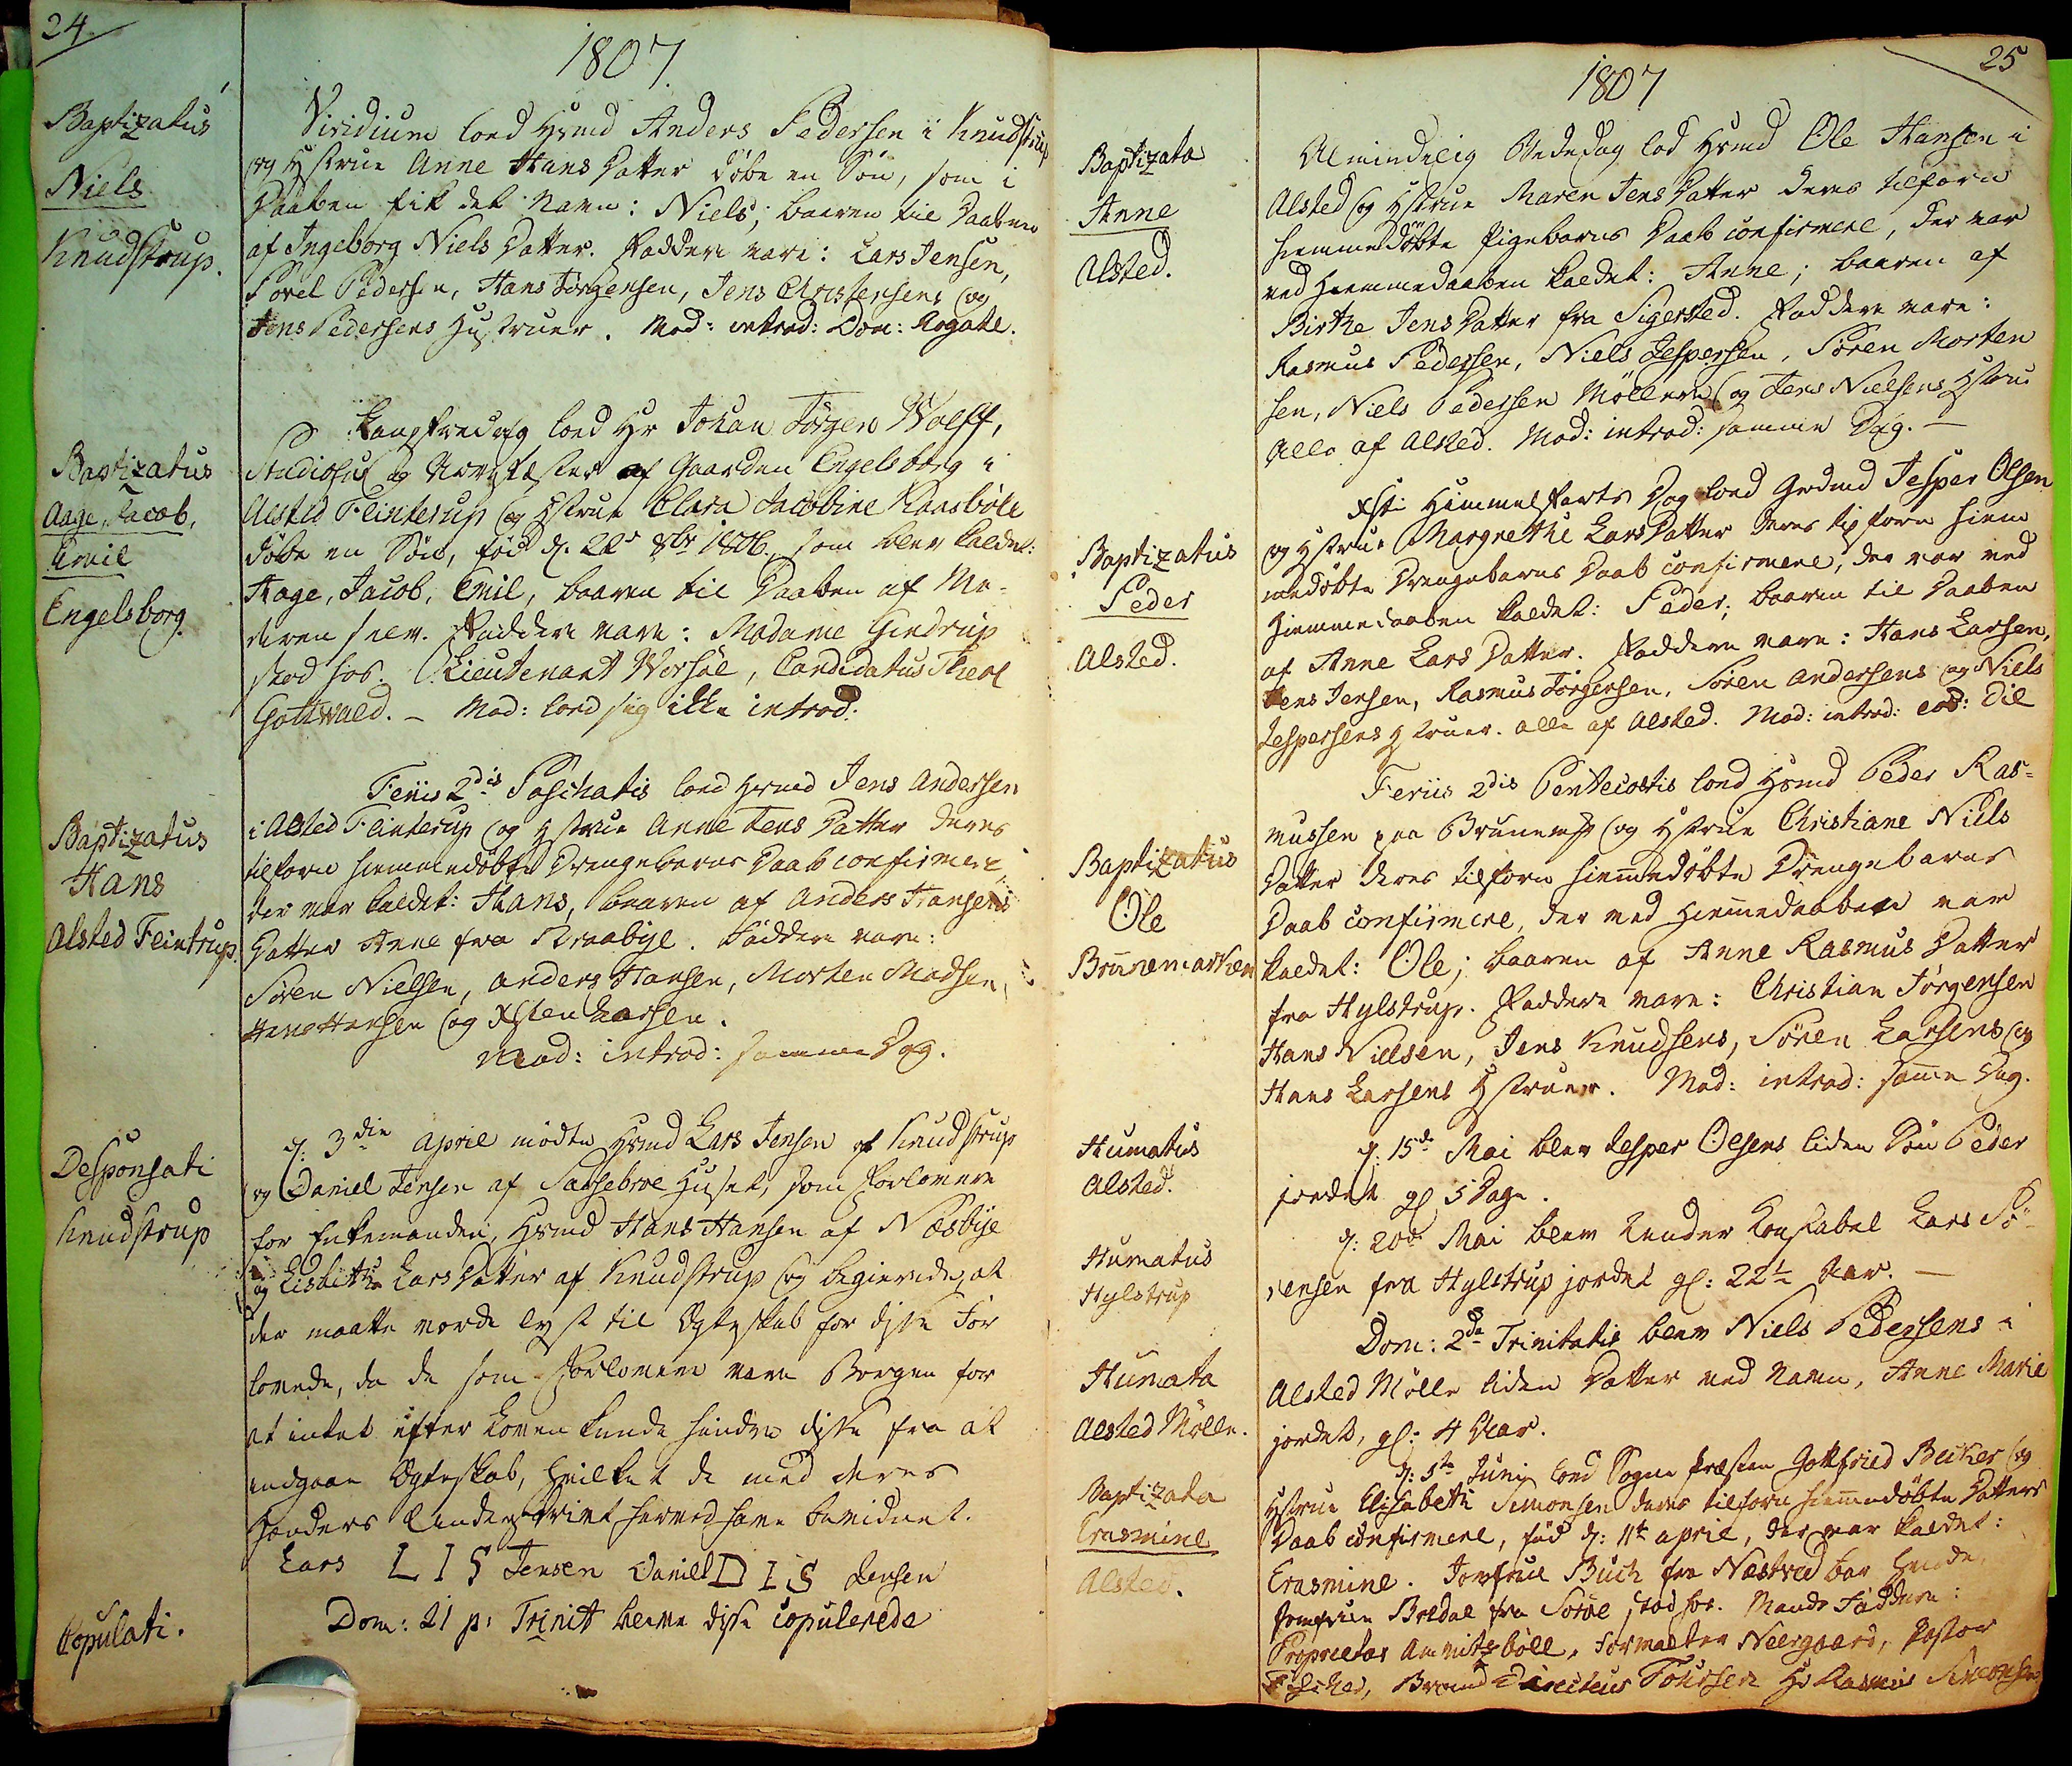24.
Baptizatus
Niels
Knudstrup.
Baptizatus
Aage Jacob
Emil
Engelsborg
Baptizatus
Hans
Alsted Flinterup.
Desponsati
Knudstrup
Copulati.
1807.
Viridium loed Hsmd Anders Pedersen i Knudstrup
og Hstrue Anne Hans Datter døbe en Søn, som i
Daaben fik det Navn: Niels; baaren til Daaben
af Ingeborg Niels Datter. Faddere vare: Lars Jensen,
Povel Pedersen, Hans Jørgensen, Jens Christensens og
Jens Pedersens Hustruer. Mod: introd: Dom: Rogate.
Langfredag loed Hr Johan Jørgen Wolff,
Studiosus og Arvefæsterr af Gaarden Engelsborg i
Alsted Flinterup og Hustrue Clara Jacobine Kaasbøle
døbe en Søn, fød d: 20de 8br 1806, som blev kaldet:
Aage, Jacob Emil, baaren til Daaben af Mo¬
deren selv. Faddere vare: Madame Gindrup
stod hos. Lientenant Wersøe, Candidatus Theol
Gottwald. - Mod: loed sig ikke introd:
Feriis 2dis Paschatis loed Hsmd Jens Andersen
i Alsted Flinterup og Hstrue Anne Jens Datter deres
tilforn hiemmedøbte Drengebarns Daab confirmere
der var kaldet: Hans, baaren af Anders Hansens
Datter Anne fra Braabye. Faddere vare:
Søren Nielsen, Anders Hansen, Morten Madsen
Hans Hansen og Xsten Larsen.
Mod: introd: samme Dag.
d: 3die April mødet Hsmd Lars Jensen af Knudstrup
og Daniel Jensen af Sa##sebroe Huset, som Forlovere
for Enkemanden, Hsmd Hans Hansen af Næsbye
og Lisbeth Lars Datter af Knudstrup og begierede, at
der maatte vorde lyst til Ægteskab for disse For
lovede, da de som Forlovere vare Borgen for
at intet efter Konen kunde hindre disse fra at
indgaare Ægteskab, hvilket de med deres
Hænders Underskrivt herved have bevidnet.
Lars L I S Jensen Daniel D I S Jensen
Dom: 21 p: Trinit bleve disse copulerede
Baptizata
Anne
Alsted.
Baptizatus
Peder
Alsted.
Baptizatus
Ole
Brunemarken
Humatus
Alsted.
Humatus
Hylstrup
Humata
Alsted Mølle.
Baptizata
Erasmine
Alsted.
25.
1807
Almindelig Bededag lod Hsmd Ole Hansen i
Alsted og Hustrue Maren Jens Datter deres tilforn
hiemmedøbte Pigebarns Daab confirmere, der var
ved Hiemmedaaben kaldet: Anne; baaren af
Birthe Jens Datter fra Sigersted. Faddere vare:
Rasmus Pedersen, Niels Jespersen, Søren Morten
sen, Niels Pedersen Mølleren og Jens Nielsens Hstrue
Alle af Alslev. Mod: introd: samme Dag.
Xsti Himmelfarts Dag loed Grdmd Jesper Olsen
og Hstrue Margrethe Lars Datter deres tilforn hiem
medøbte Drengebarns Daab confirmere, der var ved
Hiemmedaaben kaldet: Peder; baaren til Daaben
af Anne Lars Datter. Faddere vare: Hans Larsen,
Jens Jensen, Rasmus Jørgensen, Søren Andersens og Niels
Jespersens Hstruer. alle af Alsted. Mod: introd: eod: Die
Feriis 2dis Pentecostis loed Hsmd Peder Ras¬
mussen paa Brunem## og Hstrue Christiane Niels
Datter deres tilforn hiemmedøbte Drengebarns
Daab confirmere, der ved Hiemmedaaben var
kaldet: Ole; baaren af Anne Rasmus Datter
fra Hylstrup. Faddere vare: Christian Jørgensen
Hans Nielsen, Jens Knudsens, Søren Larsens og
Hans Larsens Hstruer. Mod: introd: samme Dag.
d; 15de Mai blev Jesper Olsens liden Søn Peder
joder gl 5 Dage.
d: 20de Mai blev Under Konstabel Lars Sø¬
rensen fra Hylstrup jordet gl: 22½ Aar.
Dom: 2da Trinitatis blev Niels Pedersens i
Alsted Mølle liden Datter ved Navn, Anne Marie
jordet gl: 4 Aar.
d: 5te Juni loed Sogne Præsten Gottfried Becher og
Hstrue Elisabeth Simonsen deres tilforn hiemmedøbte Datters
Daab confirmere, fød d: 11te April, der var kaldet:
Erasmine. Jomfrue Buch fra Næstved bar hende
Jomfrue Bodel fra Sorøe stod hos.l. Mands Faddere:
Proprietar Amnitzbøll, Forvalter Neergaard, Pastor
Fischer, Br## Directeur Tohrsen Hr Rasmus Simonsen
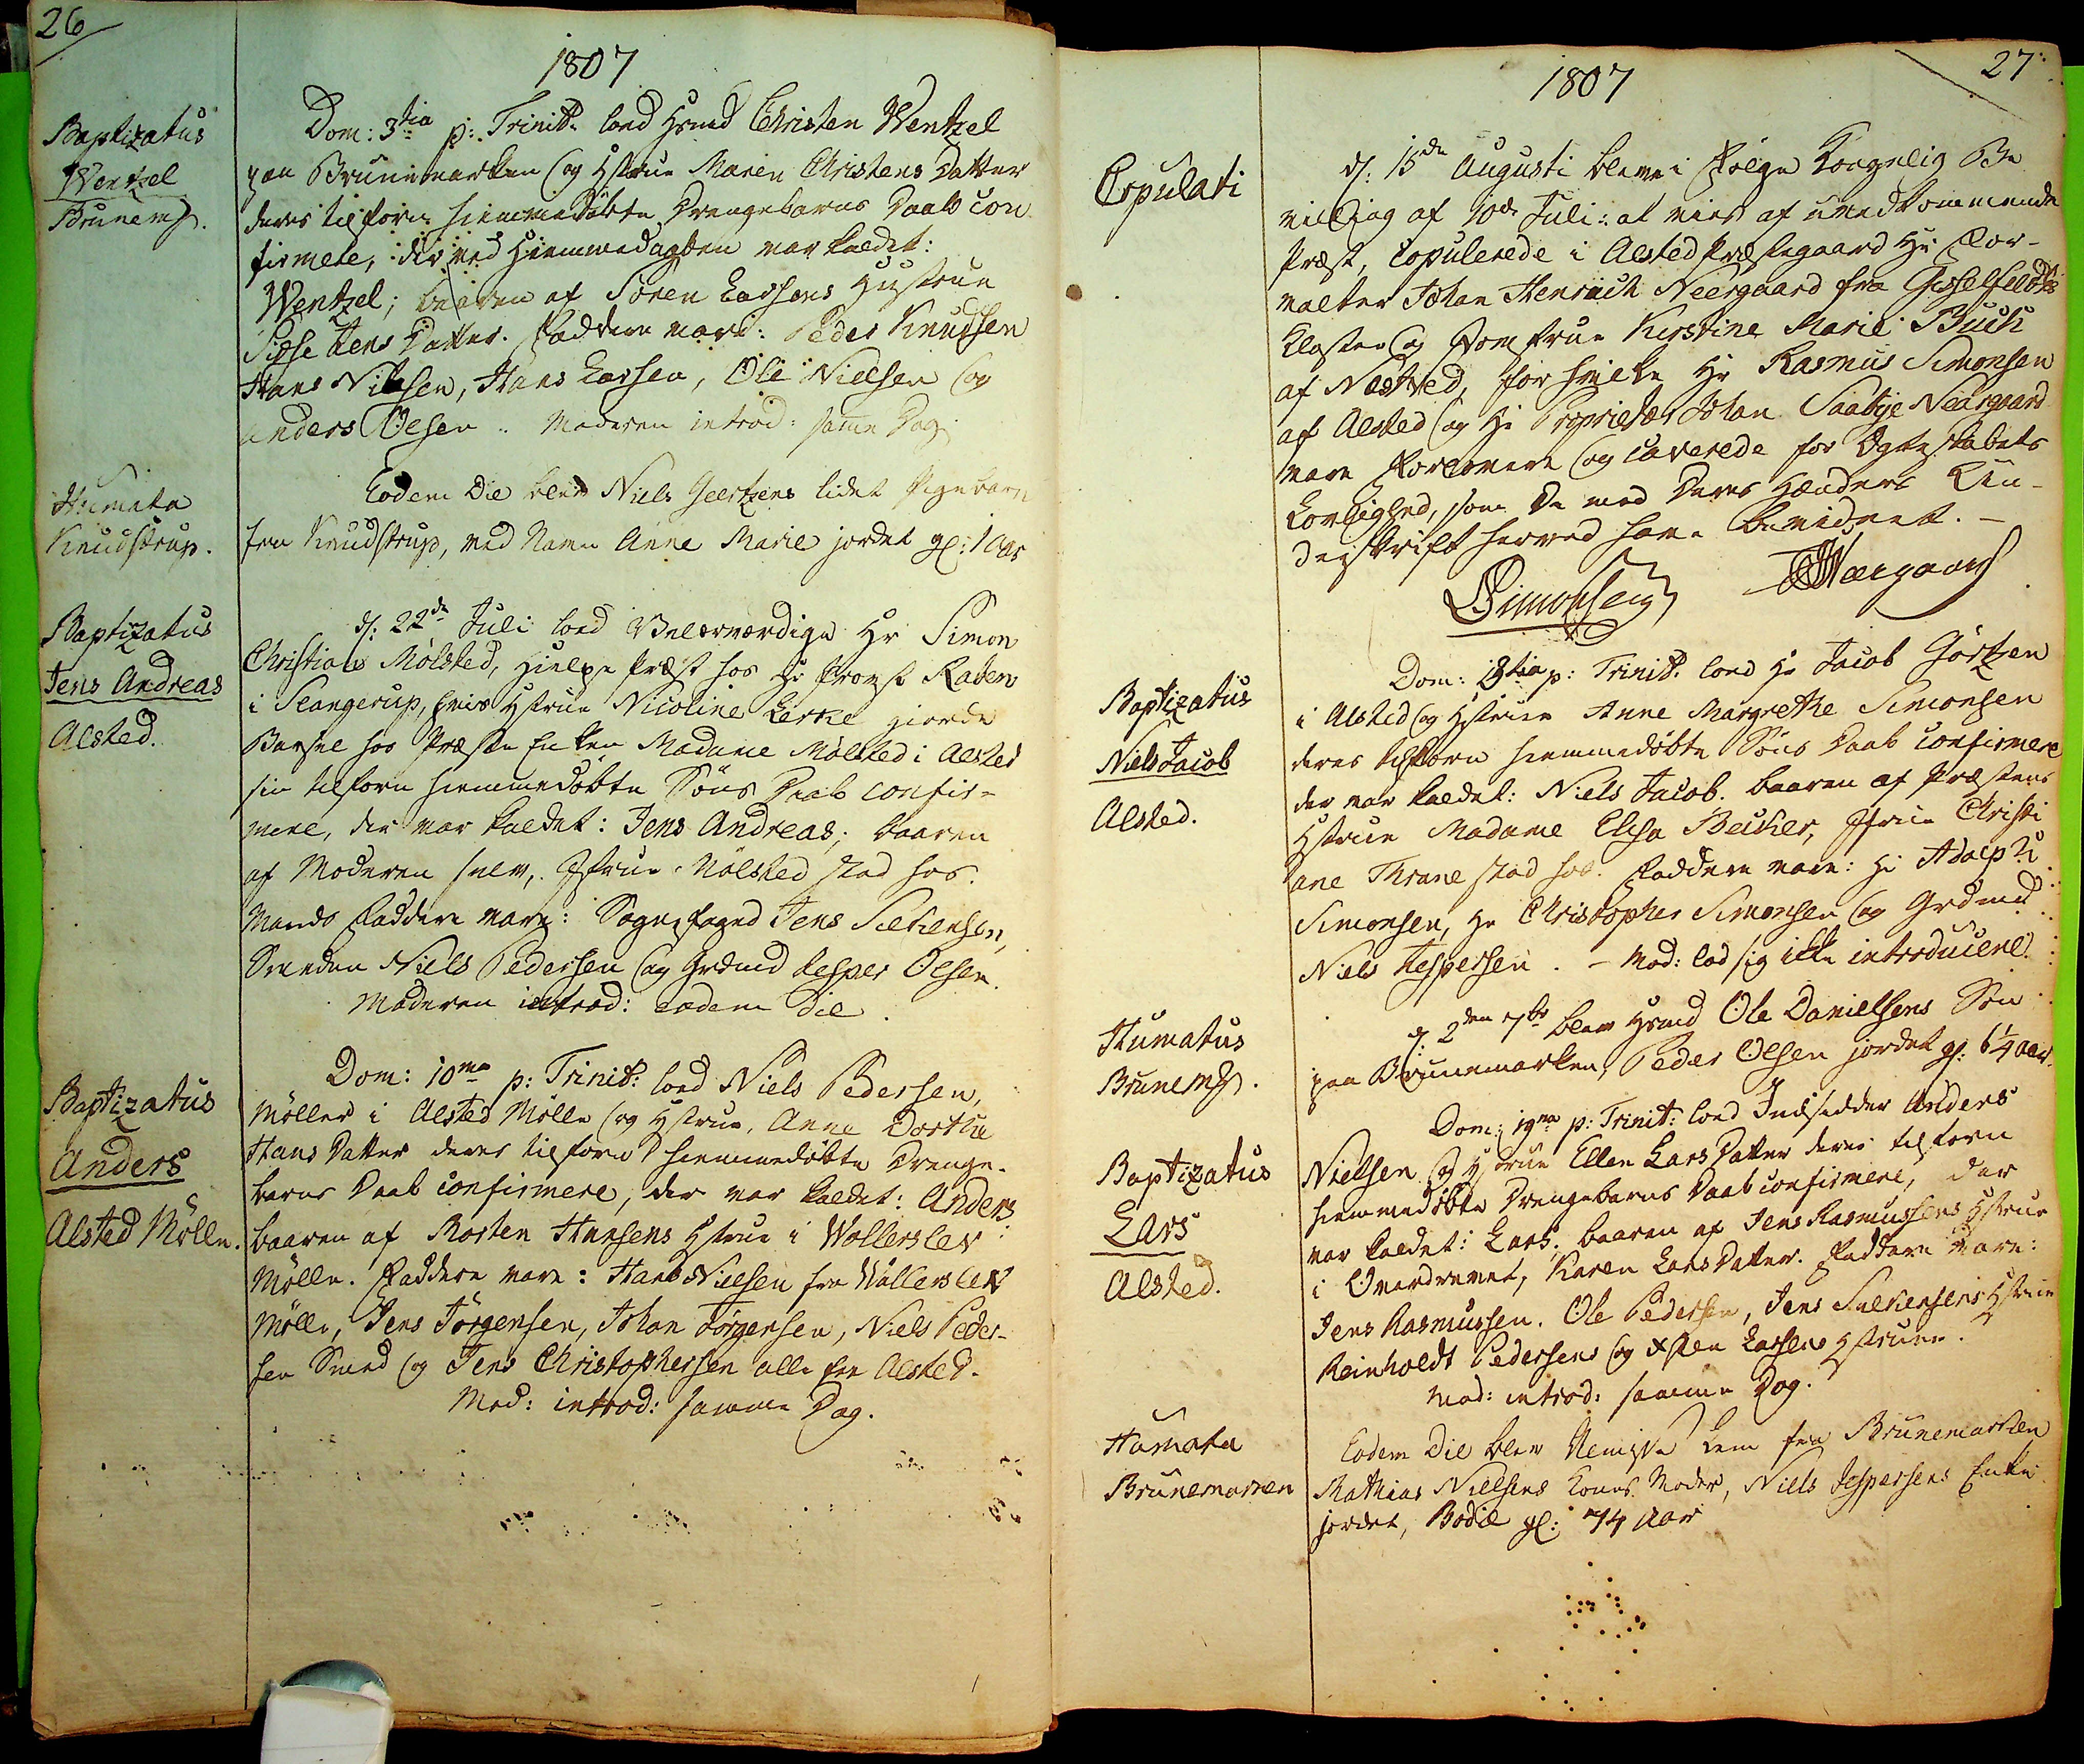26
Baptizatus
Wentzel
Brunem##.
Humata
Knmudstrup
Baptizatus.
Jens Andreas
Alsted.
Baptizatus
Anders
Alsted Mølle.
1807
Dom: 3tia p: Trinit: loed Hsmd Christen Wentzel
paa Brunemarken og Hstrue Maren Christens Datter
deres tilforn hiemmedøbte Drengebarns Daab con
firmere, der ved Hiemmedaaben var kaldet:
Wentzel; baaren af Søren Larsens Hustrue
Sidse Jens Datter. Faddere vare: Peder Knudsen
Hans Nielsen, Hans Larsen, Ole Nielsen og
Anders Olesen. Moderen introd: samme Dag
Eodem Die blev Niels Geertzens lidet Pigebarn
fra Knudstrup, ved Navn Anne Mariæ jordet gl: 1 Aar
d: 22de Juli loed Velærværdige Hr Simon
Christian Mølsted, Hielpe Præst hos Hr Provst Raben
i Slangerup, hvis Hstrue Nicoline Lerke giorde
Barsel hos Præsten Enken Madame Mølsted i Alsted
sin tilforn hiemmedøbte Søns Daab confir¬
mere, der var kaldet: Jens Andreas; baaren
af Moderen selv, Jfrue Mølsted stod hos
Mands Faddere vare: Sognefoged Jens Silkensen,
Smeden Niels Pedersen og Grdmd Jesper Olsen
Moderen introd: eodem Die
Dom: 10ma p: Trinit: loed Niels Pedersen
Møller i Alsted Mølle og Hustrue, Anne Dorthe
Hans Datter deres tilforn hiemmedøbte Drenge¬
barns Daab confirmere, der var kaldet: Anders
baaren af Morten Hansens Hstrue i Wollerslev
Mølle. Faddere vare: Hans Nielsen fra Wollerslev
Mølle, Jens Jørgensen, Johan Jørgensen, Niels Peder¬
sen Smed og Jens Christophersen alle fra Alsted.
Mod: introd: samme Dag.
Copulati
Baptizatus
Niels Jacob
Alsted.
Humatus
Brunem##.
Baptizatus
Lars
Alsted.
Humata
Brunemarken
27
1807
d: 15de Augusti bleve i Følge Kongelig Be
villing af 10de Juli: at vies af uvedkommende
Præst copulerede i Alsted Præstegaard Hr For¬
valter Johan Henrich Neergaard fra Gisselfelds
Kloster og Jomfrue Kirstine Marie Buch
af Næsted, for hvilke Hr Rasmus Simonsen
af Alsted og Hr Proprietær Johan Saabye Neergaard
vare Forlovere og loverede for Ægteskabets
Lovlighed, som de med deres Hænders Un-
derskrift herved have bevidnet
Simonsen Neeergaard
Dom: 13tia p: Trinit: loed Hr Jacob Gørtzen
i Alsted og Hstrue Anne Margrethe Simonsen
deres tilforn hiemmedøbte Søns Daab confirmeret
der var kaldet: Niels Jacob. baaren af Præstens
Hstrue Madame Elisa Becker, Jfrue Christi
ane Thrane stod hos. Faddere vare: Hr Adolph
Simonsen, Hr Christopher Simonsen og Grdmd
Niels Jespersen . - Mod: lod sig ikke introducere.
d: 2den blev Hsmd Ole Danielsens Søn
paa Brunemarken, Peder Olsen jordet gl: 6¼ Aar.
Dom: 19na p: Trinit: loed Indsidder Anders
Nielsen og Hstrue Ellen Lars Datter deres tilforn
hiemmedøbte Drengebarns Daab confirmere, der
var kaldet: Lars. baaren af Jens Rasmussens Hstrue
i Overdrevet, Karen Lars Datter. Faddere vare:
Jens Rasmussen. Ole Pedersen, Jens Silkensens Hustrue
Reinholdt Pedersens og Xsten Larsens Hstruer
Mod: introd: samme Dag.
Eodem Die blev Almisse Lem fra Brunemarken
Mathias Nielsens Kones Moder, Niels Jespersens Enke
jordet Bodil gl: 74 Aar